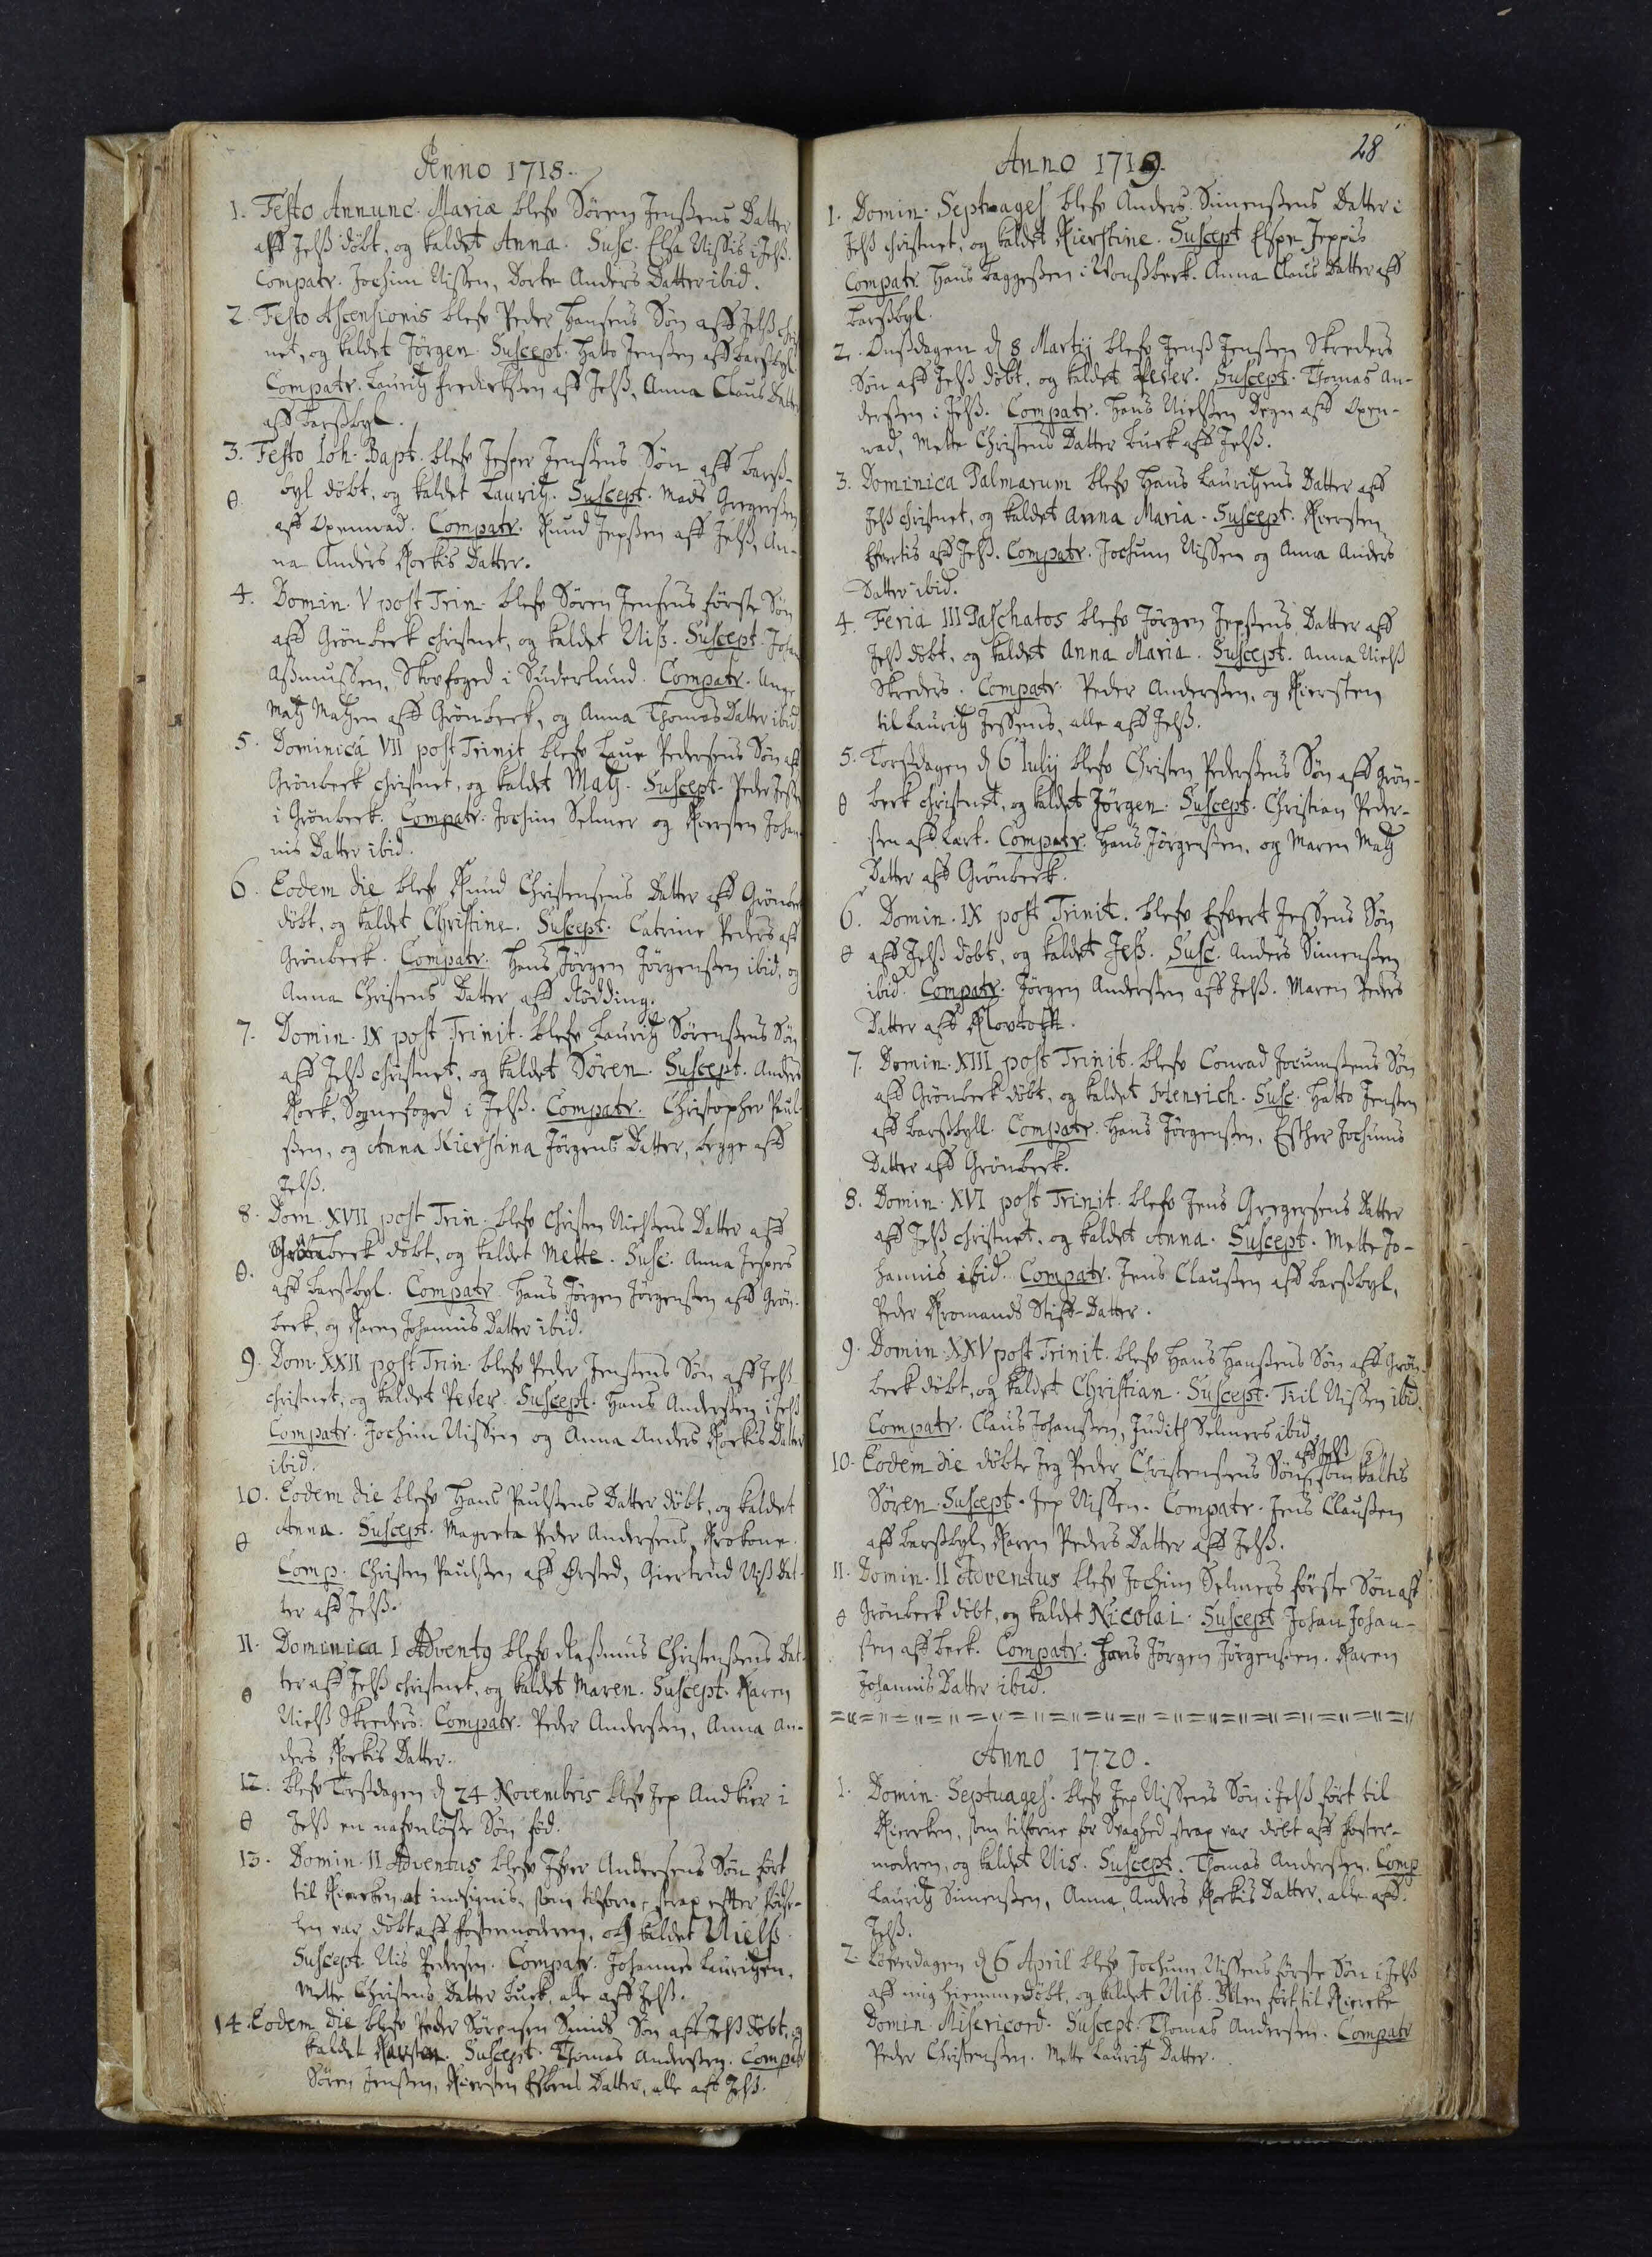Anno 1718.
1. Festo Annunc. Mariæ blefv Søren Jenssens Datter
aff Jelss døbt, og kaldet Anna. Susc. Elsa Nissis i Jelss.
Compatr. Jochim Nissen, Dorthe Anders Datter ibid.
2. Festo Ascensionis blefv Peder Hansens Søn aff Jelss chris
net, og kaldet Jørgen. Suscept. Hatto Jenssen aff Barssbyl.
Compatr. Lauritz Fredicksen aff Jelss, Anna Claus Datter
aff Barssbyl.
3. Festo Ioh. Bapt. blefv Jesper Jensens Søn aff Barss¬
byl døbt, og kaldet Lauritz . Suscept. Mads Gregerssen
aff Oxemvad. Compatr. Knud Jepssen aff Jelss, An¬
na Anders Kockis Datter.
4. Domin. V post Trin. blefv Søren Jensens første Søn
aff Grønbeck christnet, og kaldet Niss. Suscept. Johan
Assmussen, Skovfoged i Suderlund. Compatr. Unge
Matz Matzen aff Grønbeck, og Anna Thomas Datter ibid.
5. Dominica VII post Trinit blefv Laue Pedersens Søn aff
Grønbeck christnet, og kaldet Matz. Suscept. Peder Jessen
i Grønbeck. Compatr. Jochim Selmer og Kiersten Johan¬
nis Datter ibid
6. Eodem die blefv Knud Christensens Datter aff Grønbec 
døbt, og kaldet Christine. Suscept. Catrine Peders aff
Grønbeck. Compatr. Hans Jørgen Jørgenssen ibid, og
Anna Christens Datter aff Rødding.
7. Domin. IX post Trinit. blefv Lauritz Sørenssens Søn
aff Jelss christnet, og kaldet Søren. Suscept. Anders
Kock, Sognefoged i Jelss. Compatr. Christopher Paul¬
ssen, og Anna Kierstina Jørgens Datter, begge aff
Jelss.
8. Dom. XVII post Trin blefv Christen Nielssens Datter aff
Grønbeck døbt, og kaldet Mette . Susc. Anna Jespers
aff Barssbyl. Compatr. Hans Jørgen Jørgenssen aff Grøn¬
beck, og Karen Johannis Datter ibid.
9. Dom. XXII post Trin. blefv Peder Jenssens Søn aff Jelss
christnet, og kaldet Peder. Suscept. Hans Anderssen i Jelss
Compatr. Jochim Nissen og Anna Anders Kockis Datter
ibid.
10. Eodem die blefv Hans Paulssens Datter døbt, og kaldet
Anna. Suscept. Magreta Peder Andersens, Krokone
Comp. Christen Paulssen aff Ørsted, Giertrud Niss Dat¬
ter aff Jelss.
11. Dominica I Adventq blefv Rassmus Christenssens Dat¬
ter aff Jelss christnet, og kaldet Maren. Suscept. Karen
Nielss Skreders. Compatr. Peder Anderssen, Anna An¬
ders Kockis Datter.
12. blefv Torssdagen d 24 Bovembris blefv Jep Andkier i
Jelss en nafvnløsse Søn fød
13. Domin. II Adventus blefv Ifver Andersens Søn ført
til Kiercken at indsignis, som tilforne strax efter Føds¬
len var døbt aff Fostermoderen, og kaldet Nielss.
Suscept. Nis Pedersen. Compatr. Johannes Lauritzen.
Mette Christens Datter Buck, alle aff Jelss.
14. Eodem die blefv Peder Sørensen Smids Søn aff Jelss døbt, og
kaldet Karsten. Suscept. Thomas Anderssen. Comp##
Søren Jenssen, Kiersten Esbens Datter, alle aff Jelss.
28
Anno 1719.
1. Domin. Septvages. blefv Anders Simenssens Datter i
Jelss christnet, og kaldet Kierstine. Suscept Elspe Jeppis
Compatr. Hans Baggessen i Vonssbeck. Anna Claus Datter aff
Barssbyl.
2. Onsdagen d 8 Martij blefv Jenss Jenssen Skreders
Søn aff Jelss døbt, og kaldet Peder. Suscept. Thomas An¬
dersen i Jelss. Compatr. Hans Nielssen Degn aff Oxen¬
vad, Mette Christens Datter Buck aff Jelss.
3. Dominica Palmarum blefv Hans Lauritzens Datter aff
Jelss christnet, og kaldet Anna Maria. Suscept. Kiersten
Efvertis aff Jelss. Compatr. Jochum Nissen og Anna Anders
Datter ibid.
4. Feria III Paschatos blefv Jørgen Jepssens Datter aff
Jelss døbt, og kaldet Anna Maria. Suscept. Anna Nielss
Skreders. Compatr. Peder Anderssen, og Kiersten
til Lauritz Jessens, alle aff Jelss.
5. Torsdagen d 6 Iulij blefv Christen Pederssens Søn aff Grøn¬
beck christnet, og kaldet Jørgen . Suscept. Christian Peder¬
sen aff Lart. Compatr. Hans Jørgenssen, og Maren Matz
Datter aff Grønbeck.
6. Domin. IX post Trinit. blefv Efvert Jessens Søn
aff Jelss døbt, og kaldet Jess. Susc. Anders Simenssen
ibid. Compatr. Jørgen Andersen aff Jelss. Maren Peders
Datter aff Klovtofft.
7. Domin. XIII post Trinit. blefv Conrad Jocumssens Søn
aff Grønbeck døbt, og kaldet Henrich. Susc. Hatto Jenssen
aff Barssbyll. Compatr. Hans Jørgensen. Esther Jochums
Datter aff Grønbeck.
8. Domin. XVI post Trinit. blefv Jens Gregersens Datter
aff Jelss christnet, og kaldet Anna. Suscept. Mette Jo¬
hannis ibid. Compatr. Jens Claussen aff Barssbyl,
Peder Kromands Stiff-Datter.
9. Domin. XXV post Trinit. blefv Hans Hanssens Søn aff Grøn¬
beck døbt, og kaldet Christian. Suscept. Tiil Nissen ibid.
Compatr. Claus Johanssen, Judith Selmers ibid
aff Jelss
10. Eodem die døbte Jeg Peder Christensens Søn, som kaltis
Søren. Suscept. Jep Nissen. Compatr. Jens Claussen
aff Barssbyl, Karen Peders Datter aff Jelss.
11. Domin. II Adventus blefv Jochim Selmers første Søn aff
Grønbeck døbt, og kaldet Nicolai . Suscept. Johan Johan¬
sen aff Beck. Compatr. Hans Jørgen Jørgensen. Karen
Johannis Datter ibid.
Anno 1720.
1. Domin. Septuages. blefv Jep Nissens Søn i Jelss ført til
Kiercken, som tilforne for Svaghed strax var døbt aff Foster¬
moderen, og kaldet Nis. Suscept. Thomas Anderssen. Comp
Lauritz Simenssen, Anna Anders Kockis Datter, alle aff
Jelss.
2. Løverdagen d 6 April blefv Jochum Nissens første Søn i Jelss
aff mig hiemmedøbt, og kaldet Niss. Men ført til Kiercke
Domin. Misericord. Suscept. Thomas Anderssen. Compatr
Peder Christenssen. Mette Lauritz Datter.